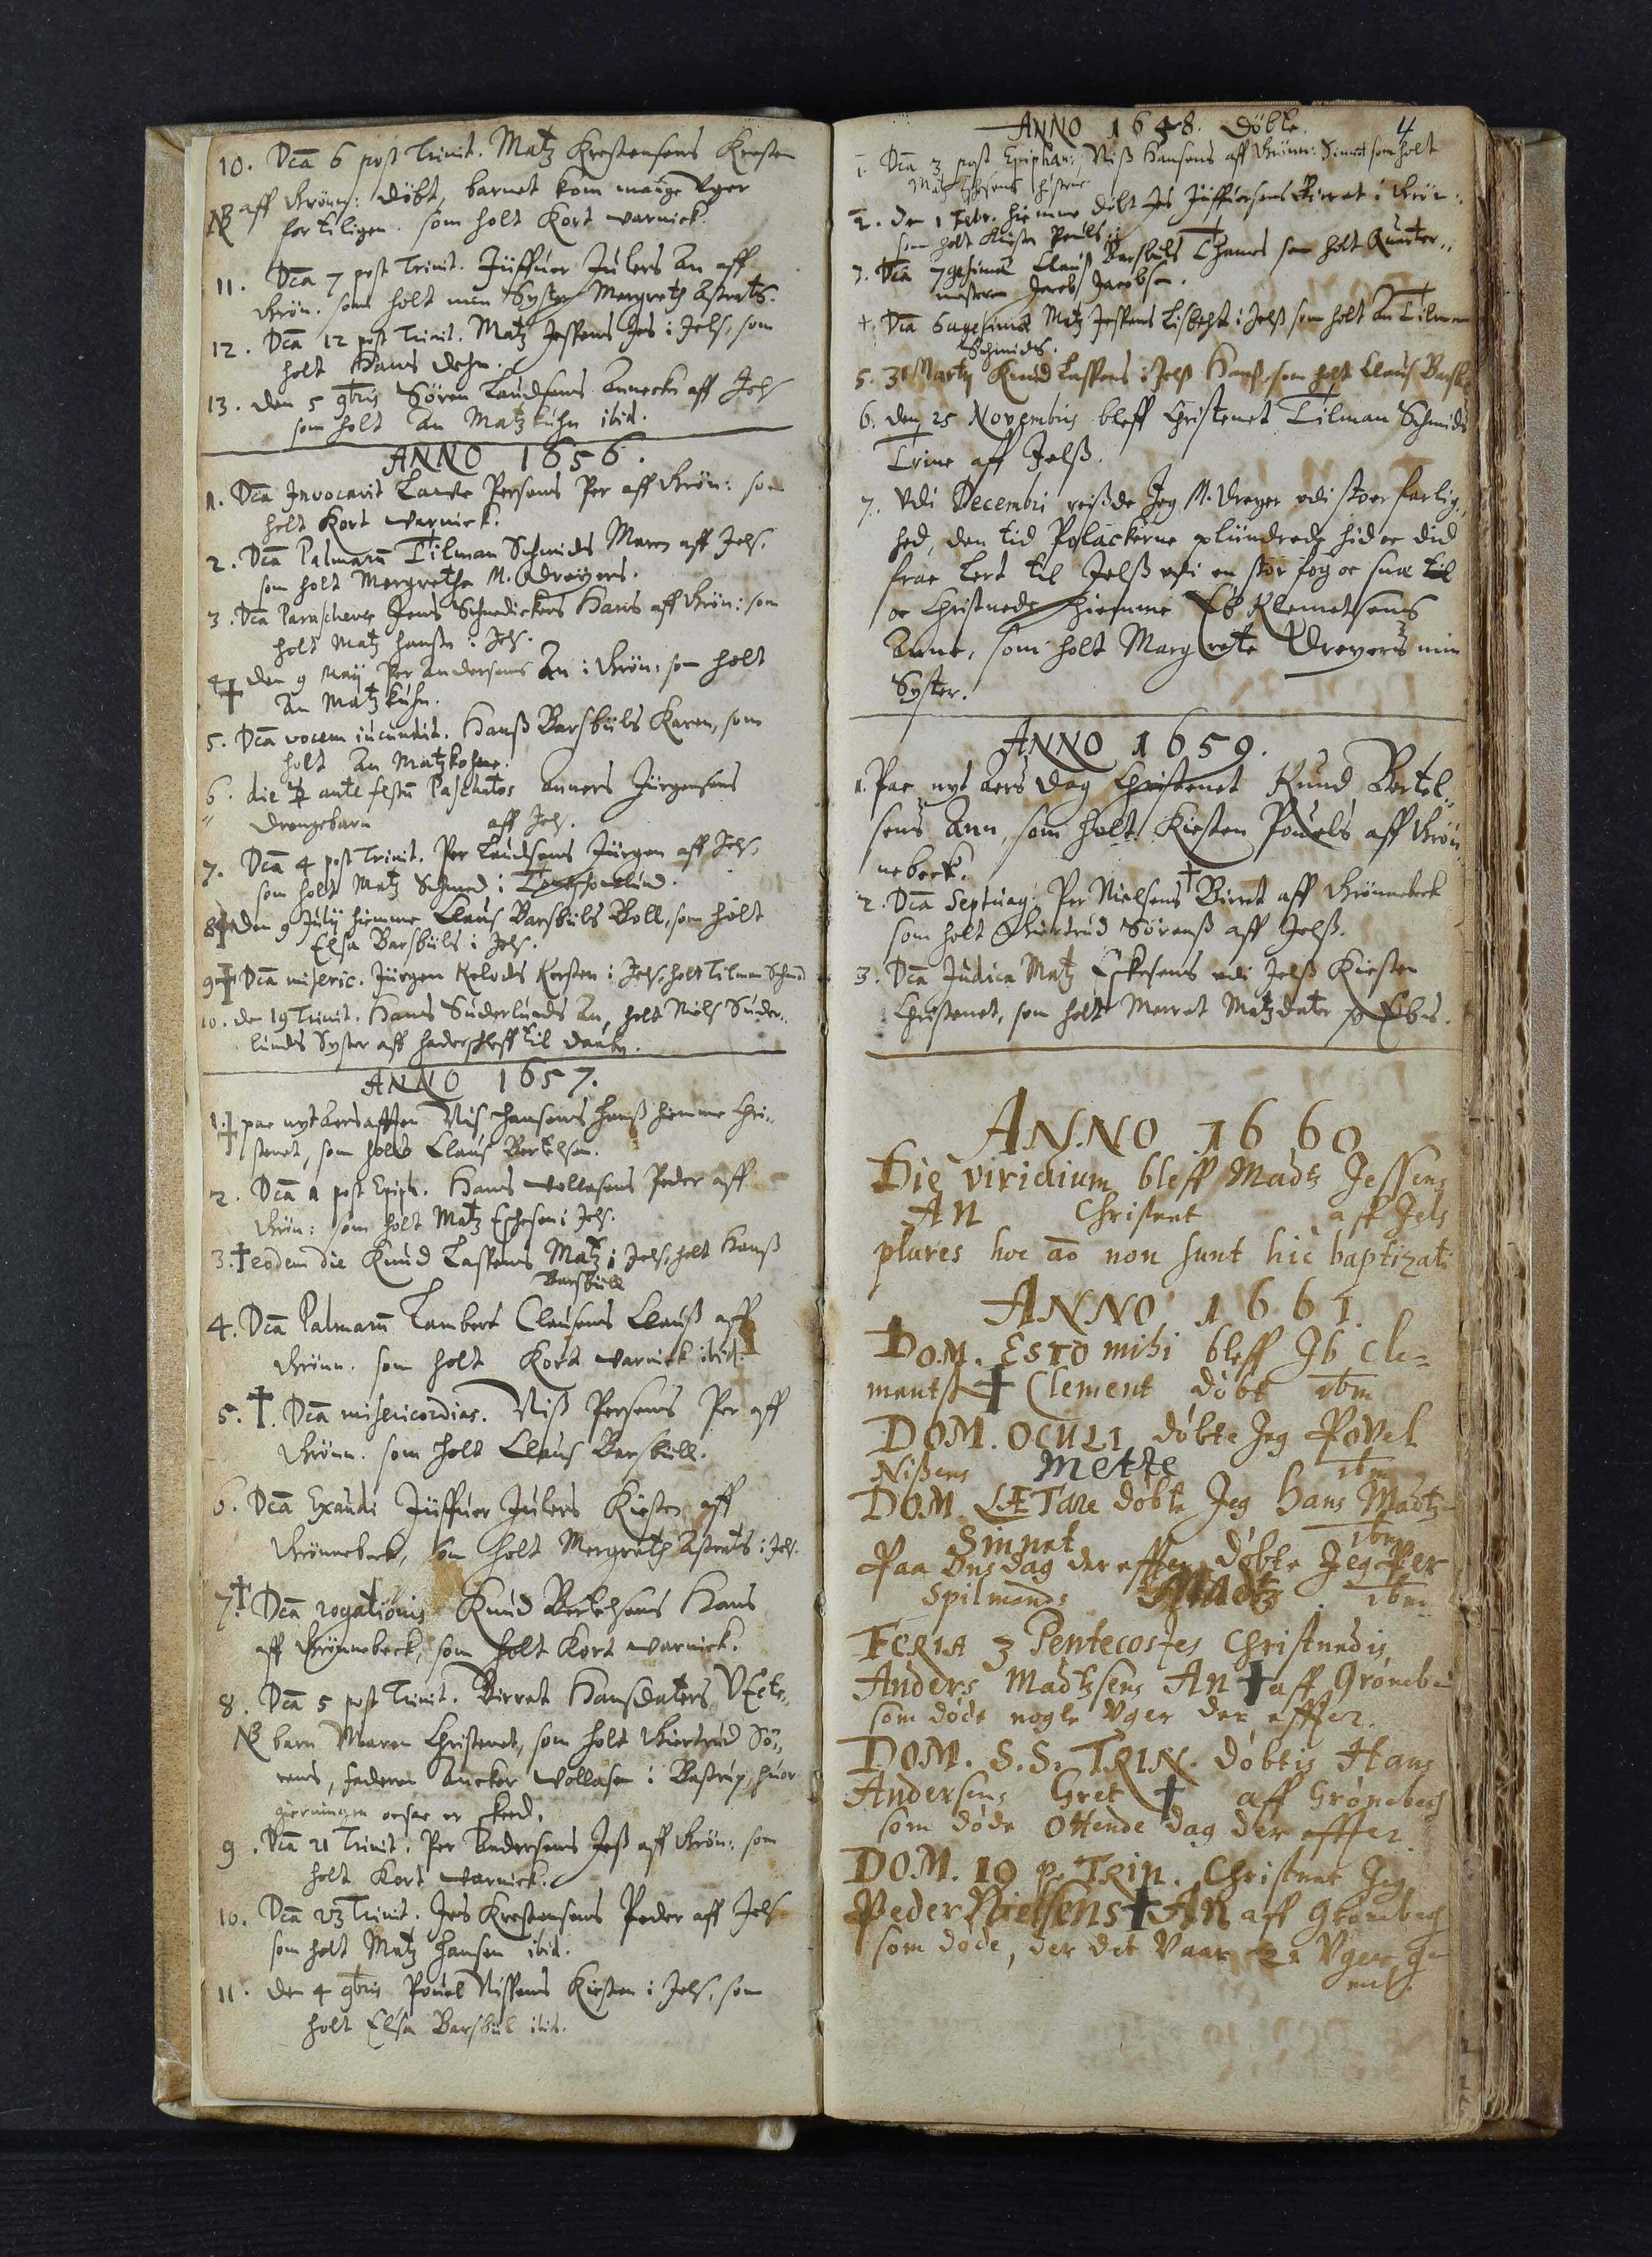10. Dca 6 post Trinit. Matz Krestensens Kresten
aff Grønn: døbt, barnet kom mange Vger
NB for tiligen. som holt Kort Varnick.
11. Dca 7 post Trinit. Juffuer Julers An aff
Grøn. som holt min Syster Mergreth Astrats.
12. Dca 12 post Trinit. Matz Jessens Jens i Jels, som
holt Hans Dehn.
13. Den 5 9tris Søren Laudsens Annecke aff Jels
som holt An Matz Kuhn ibid.
ANNO 1656.
1. Dca Invocavit Lave Persens Per aff Grøn: som
holt Kort Varnick.
2. Dca Palmaru Tilman Schmids Maren aff Jels,
som holt Mergrethe M. Dreyers.
3. Dca Parascheves Jens Schnedickers Hans aff Grøn: som
holt Matz Hansen i Jels.
4. † Den 9 Maij Per Andersens An i Grøn: som holt
An Matz Kuhn.
5. Dca vocem iucundis. Hans Barsbuls Karen, som
holt An Matz Kohne.
6. die ☿ ante festu Paschatos Anners Jurgensens
drengebarn
aff Jels.
7. Dca 4 post Trinit. Per Laudsens Jurgen aff Jels,
som holt Matz Schmed i Tovschovlund.
8 † Den 9 Julij hiemme Claus Barsbuls Boll, som holt
Elsa Barsbuls i Jels.
9 † Dca miseric. Jurgen Kelods Kersten i Jels, holt Tilman Schmed
10. Den 19 Trinit. Hans Suderlunds An, holt Niels Suder¬
lunds Syster aff Hadersleff til Daabn
ANNO 1657
1. † paa nyt Aars aften Nis Hansens Hans hiemme chri¬
stenet, som holt Claus Bertelsen
2. Dca 1 post Epiph. Hans Vollesens Peder aff
Grøn: som holt Matz Eshesen i Jels.
3. † eodem die Knud Lassens Matz i Jels, holt Hans
Barsbull
4. Dca Palmaru Lambert Clausens Claus aff
Grønn. som holt Kort Varnick ibid.
5. Dca misericordias. Nis Persens Per aff
Grønn. Som holt Claus Barsbull.
6. Dca Exaudi Juffuer Julers Kirsten aff
Grønnebeck, som holt Mergreth Astrats i Jels.
7. † Dca rogationis Knud Bertelsens Hans
aff Grønnebeck, som holt Kort Varnick.
8. Dca 5 post Trinit. Birret HansDater VEcte
NB barn Maren Christenet, som holt Giertrud Sø¬
rens, faderen Ancker Vollasen i Bastrup huer
gierningen ocsaa er skeed.
9. Dca 21 Trinit. Per Andersens Jes aff Grøn: som
holt Kort Varnick.
10. Dca 23 Trinit. Jes Krestensens Peder aff Jels
som holt Matz Hansen ibid.
11. Den 4 9tris Pouel Nissens Kirsten i Jels, som
holt Elsa Barsbul ibid.
4
ANNO 1658. Døbte
1. Dca 3 post Epiphan: Nis Hansens aff Grønn: Sinnet som holt
Matz Eshesens hustrue
2. Den 1 Febr. hiemme døbt Jes Juffuersens Birret i Grøn:
som holt Kirsten Pouels.
3. Dca 7gesima Claus Barsbulls Thames som holt Quarter-
mestern Jacob Jacobsen
4. Dca 6agesima Matz Jessens Lisbeht i Jels som holt An Tilman
Schmid
5. 31 Martij Knud Lassens i Jels Hans som holt Claus Barsbul
6. Den 25 Novembris bleff Christenet Tilman Schmids
Trine aff Jels.
7. Vdi decembri reisde Jeg M. Dreyer vdi stor farlig¬
hed, den tid Polackerne plündrede hid oc did
frae Lert til Jels vdi en stor fog oc snæ til
oc Christnede hiemme Eb Klemetsens
Anne, som holt Margrete Dreyers min
Syster.
ANNO 1659.
1. Paa nyt Aars Dag Christenet Knud Bertel¬
sens Ann, som holt Kirsten Pouels aff Grøn¬
nebeck.
2. Dca Septuag. Per Nielsens Birret aff Grønnebeck
som holt Giertrud Sørens aff Jels.
3. Dca Judica Matz Eskesens vdi Jels Kirsten
Christenet, som holt Merret Matzdater p## Ebs##.
ANNO 1660
Die viridium bleff Mads Jessens
An Christenet aff Jels
plures hoc ao non sunt hic baptizati
ANNO 1661.
DOM. ESTO mihi bleff Ib Cle¬
ments † Clement døbt ibm
DOM. OCULI døbte Jeg Povel
Nissens Mette
ibm
DOM. LÆTare døbte Jeg Hans Madtze
Sinnet
ibm
Paa Onsdag der effter døbte Jeg Per
Spilmands Madtz ibm.
FERIA 3 Pentecostes Christnedis
Anders Madtzsens An † aff Grøneb
som døde nogle Vger der effter.
DOM. S. S. TRIN. Døbtis Hans
Andersens Gret † aff Grønebech
som døde ottende dag der effter.
DOM. 19 p. TRIN. Christnet Jeg
Peder Nielsens † An aff Grønebech
som døde, der det Vaar 21 Vger ga
mel.
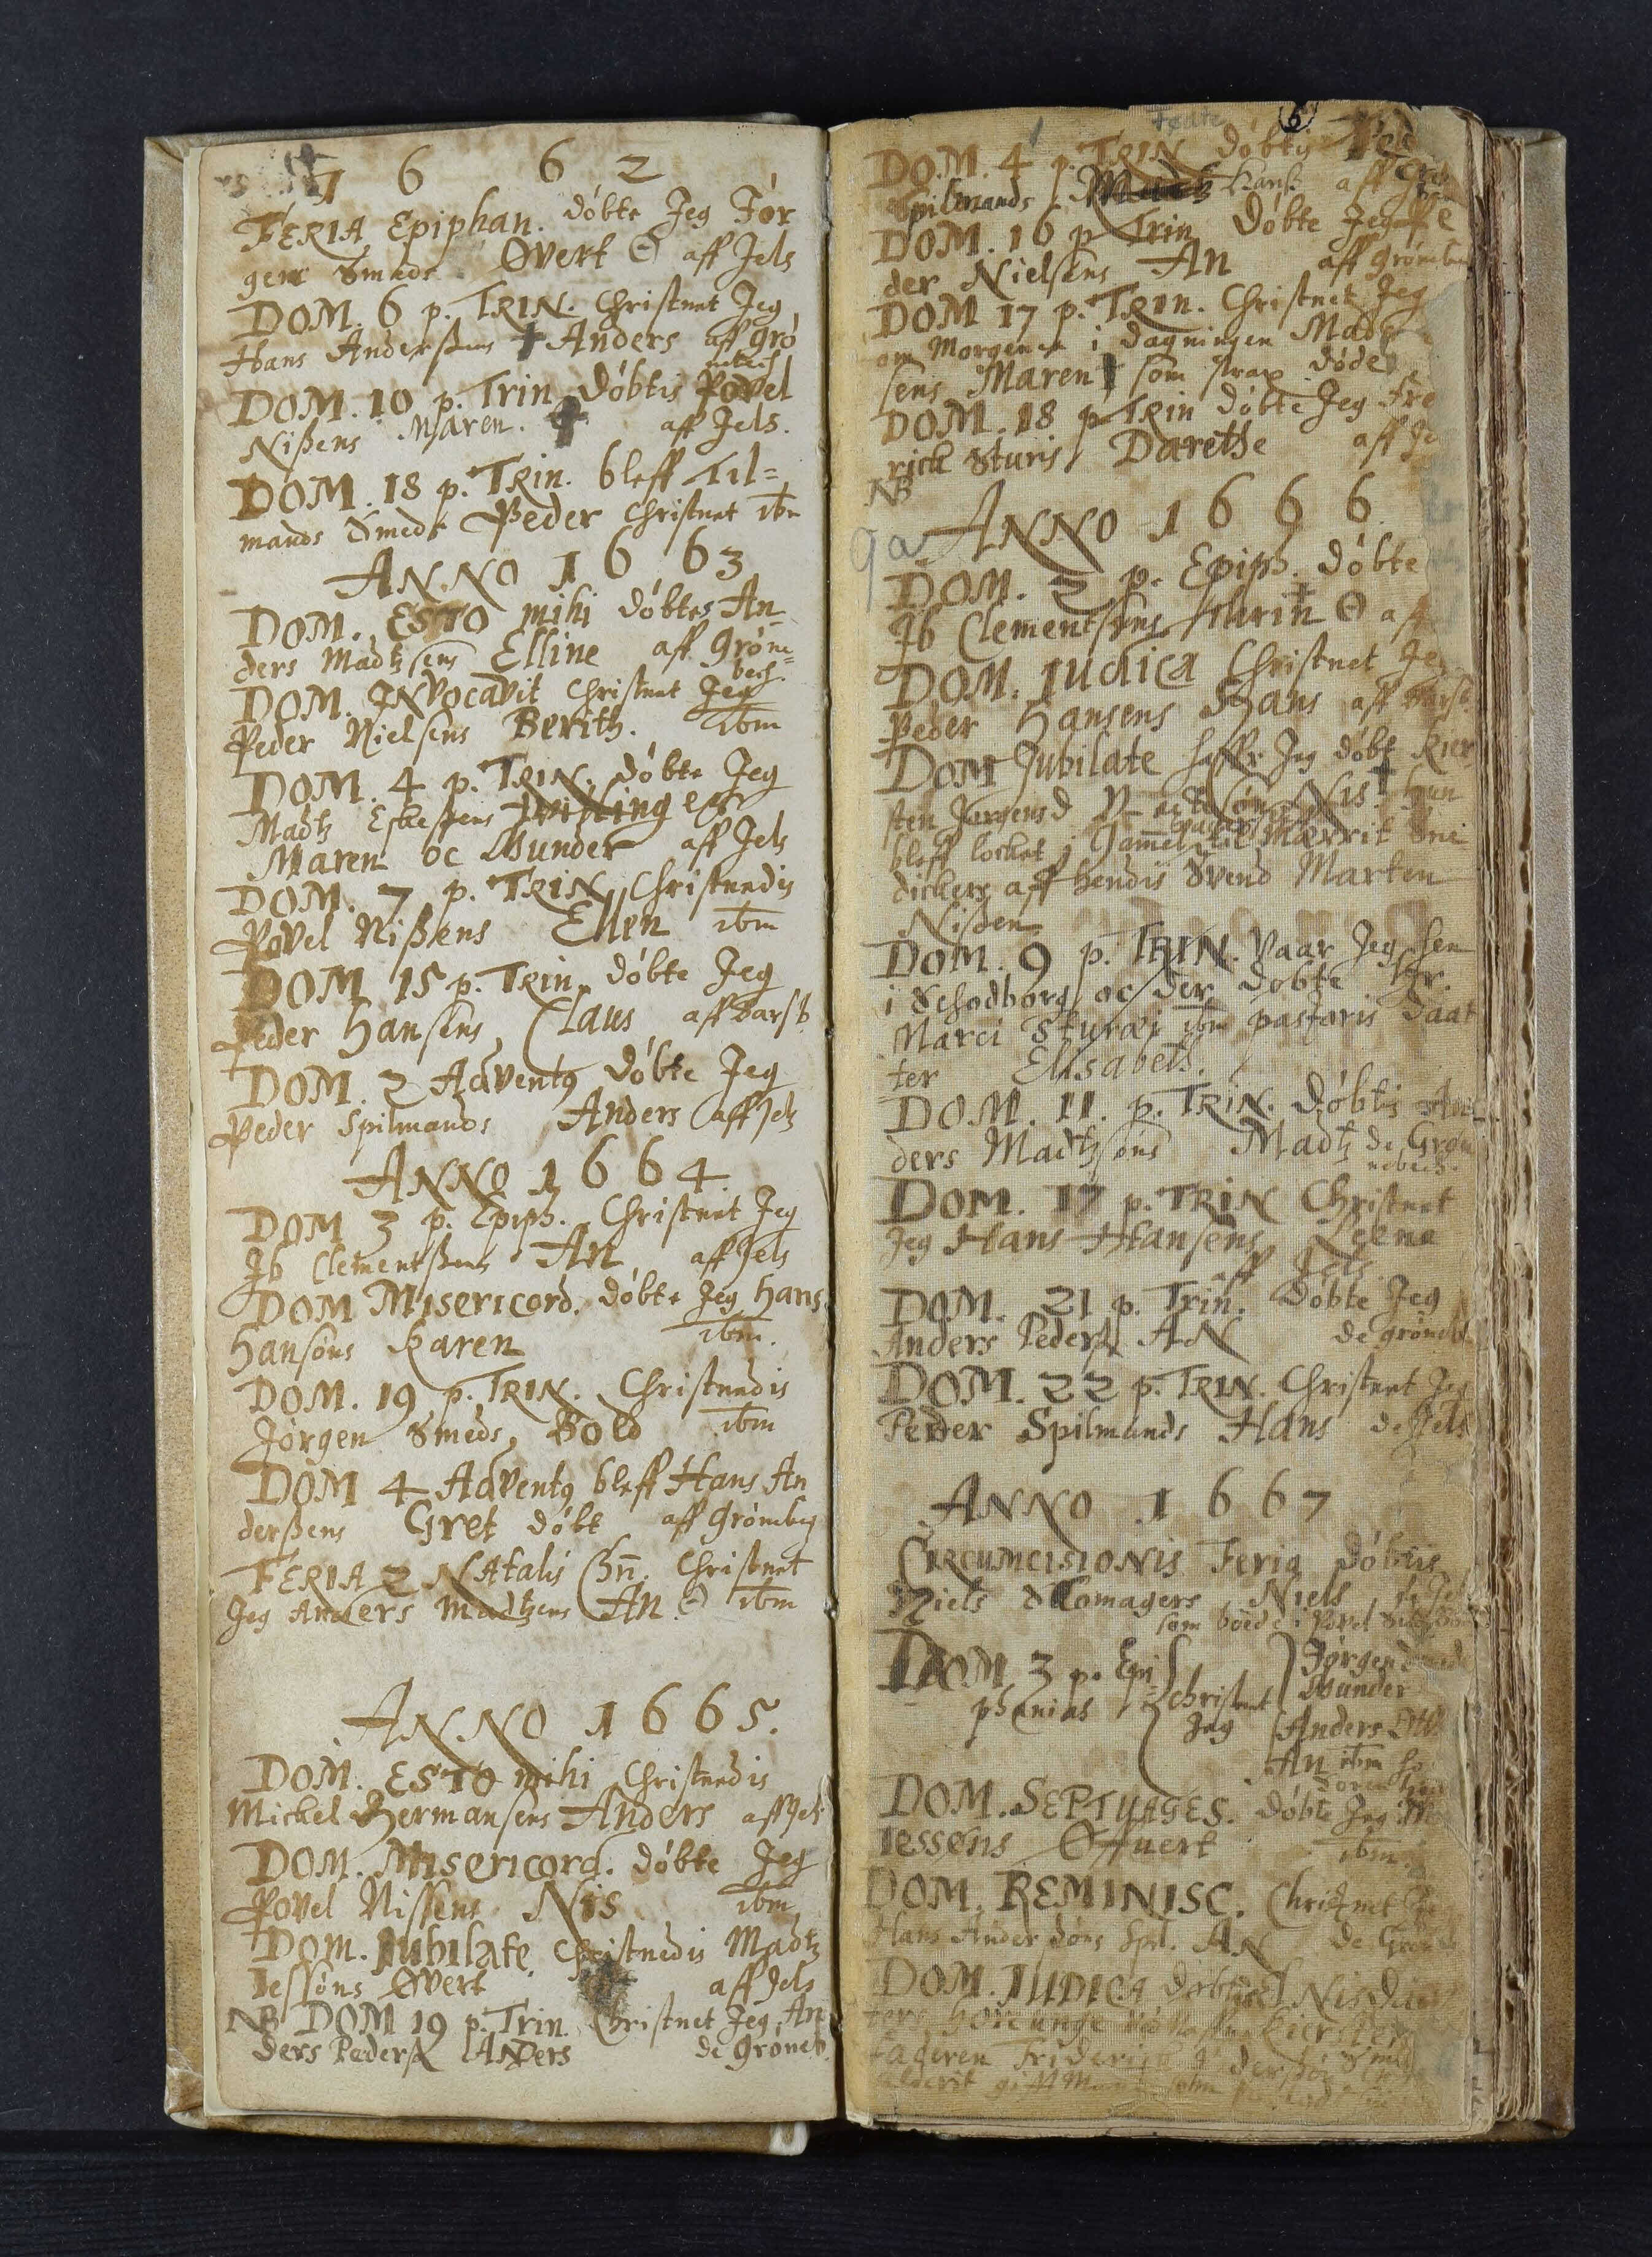1662
FERIA Epiphan. Døbte Jeg Jør
gen Smeds Øvert Θ aff Jels
DOM 6 p. TRIN. Christnet Jeg,
Hans Andersens † Anders aff Grø
nebech
DOM. 10 p. Trin Døbtes Povel
Nissens Maren
aff Jels.
DOM. 18 p. TRin. bleff Til¬
mands Smeds Peder christnet ibm.
ANNO 1663
DOM. ESTO mihi døbtes An¬
ders Madtzsens Elline aff Grøne¬
bech.
DOM. INVocavit christnet Jeg
Peder Nielsens Berith. ibm
DOM. 4 p. TRIN. døbte Jeg
Madts Eskesens tvillinger
Maren oc Gunder aff Jels
DOM. 7 p. TRIN christnedis
Povel Nisens Ellen ibm
DOM. 15 p. Ttrin. døbte Jeg
Peder Hansens Claus aff Barsb
DOM. 2 Adventq døbte Jeg
Peder Spilmands Anders aff Jels
ANNO 1664.
DOM 3 p. Epiph. Christnet jeg
Ib Clementsens An aff Jels
DOM. Misericord. Døbte Jeg Hans
Hansøns Karen
ibm.
DOM. 19 p. TRIN. christnedis
Jørgen Smeds Bold ibm
DOM. 4 Adventq bleff Hans An
dersens Gret døbt aff Grønebech
FERIA 2 Natalis Chri christnet
Jeg Anders Madtzens An Θ ibm
ANNO 1665.
DOM. ESTO mihi Christendis
Mickel Hermansens Anders aff Jels.
DOM. Misericord. Døbte Jeg
Povel Nissens NIS
ibm
DOM. Jubilate Christnedis Madtz
Jessøns Øvert
aff Jels
NB DOM 19 p. Trin. Christnet Jeg An
ders Peders Anders
de Grøneb
DOM 4 p. TRIN døbte Peder##
Spilmands ## Hans aff Gr##
DOM 16 p. Trin. døbte Jeg Pe
der Nielsens An aff Grøneb
DOM 17 p. Trin. Christnet Jeg
om Morgenen i Dagningen Madtz
sens Maren som strax døde
DOM. 18 p. Trin Døbte Jeg Fre
rick Sturis Darethe aff Je
NB
ANNO 1666
DOM. 2 p. Epiph. Døbte
Ib Clementsens Thrin Θ af
Dom. JUDICA Christnet Jeg
Peder Hansens Hans aff Barsb
Dom Jubilate hafde Jeg døbt Kier
sten JorgensD V-ecte søn Nis. Hun
##
bleff locket j Gammel til Mærrit Sni
dickers aff hendis Svend Marten
Nissen.
DOM. 9 p. TRIN. Vaar Jeg hen
i Schodborg oc der døbte Hr.
Marci Sturai ibm pastoris Daat
ter Elisabeth.
Dom. 11 p. Trin. Døbtis An
ders Madtzsøns Madtz de Grøn
nebech.
DOM. 17 p. TRIN. Christnet
Jeg Hans Hansens Leene
aff Jels.
DOM. 21 p. Trin. Døbte Jeg
Anders Peders AN de grøneb
DOM. 22 p. TRIN. Christnet Jeg
Peder Spilmands Hans de Jels
ANNO 1667
CIRCUMCISIONUS Feria døbtis
Niels Skomagers Niels
som boede I Povel
Dom 3 p. Epi
phanias
christnet
Jeg
Jørgen
Gunder
Anders Ot##
An ibm ho##
Soren ##
DOM. SEPTUAGES. Døbte Jeg
IESSØns Øffuert
ibm
DOM. REMINISC. Christnet Jeg
Hans Andersøns Spl. AN de Grøneb
DOM JIDICA Døbtis El NisDaat
ters horeunge ved Naffn Kiersten
Faderen Friderich J##derssøn ## ##
##rit gift Man## ## ## ##